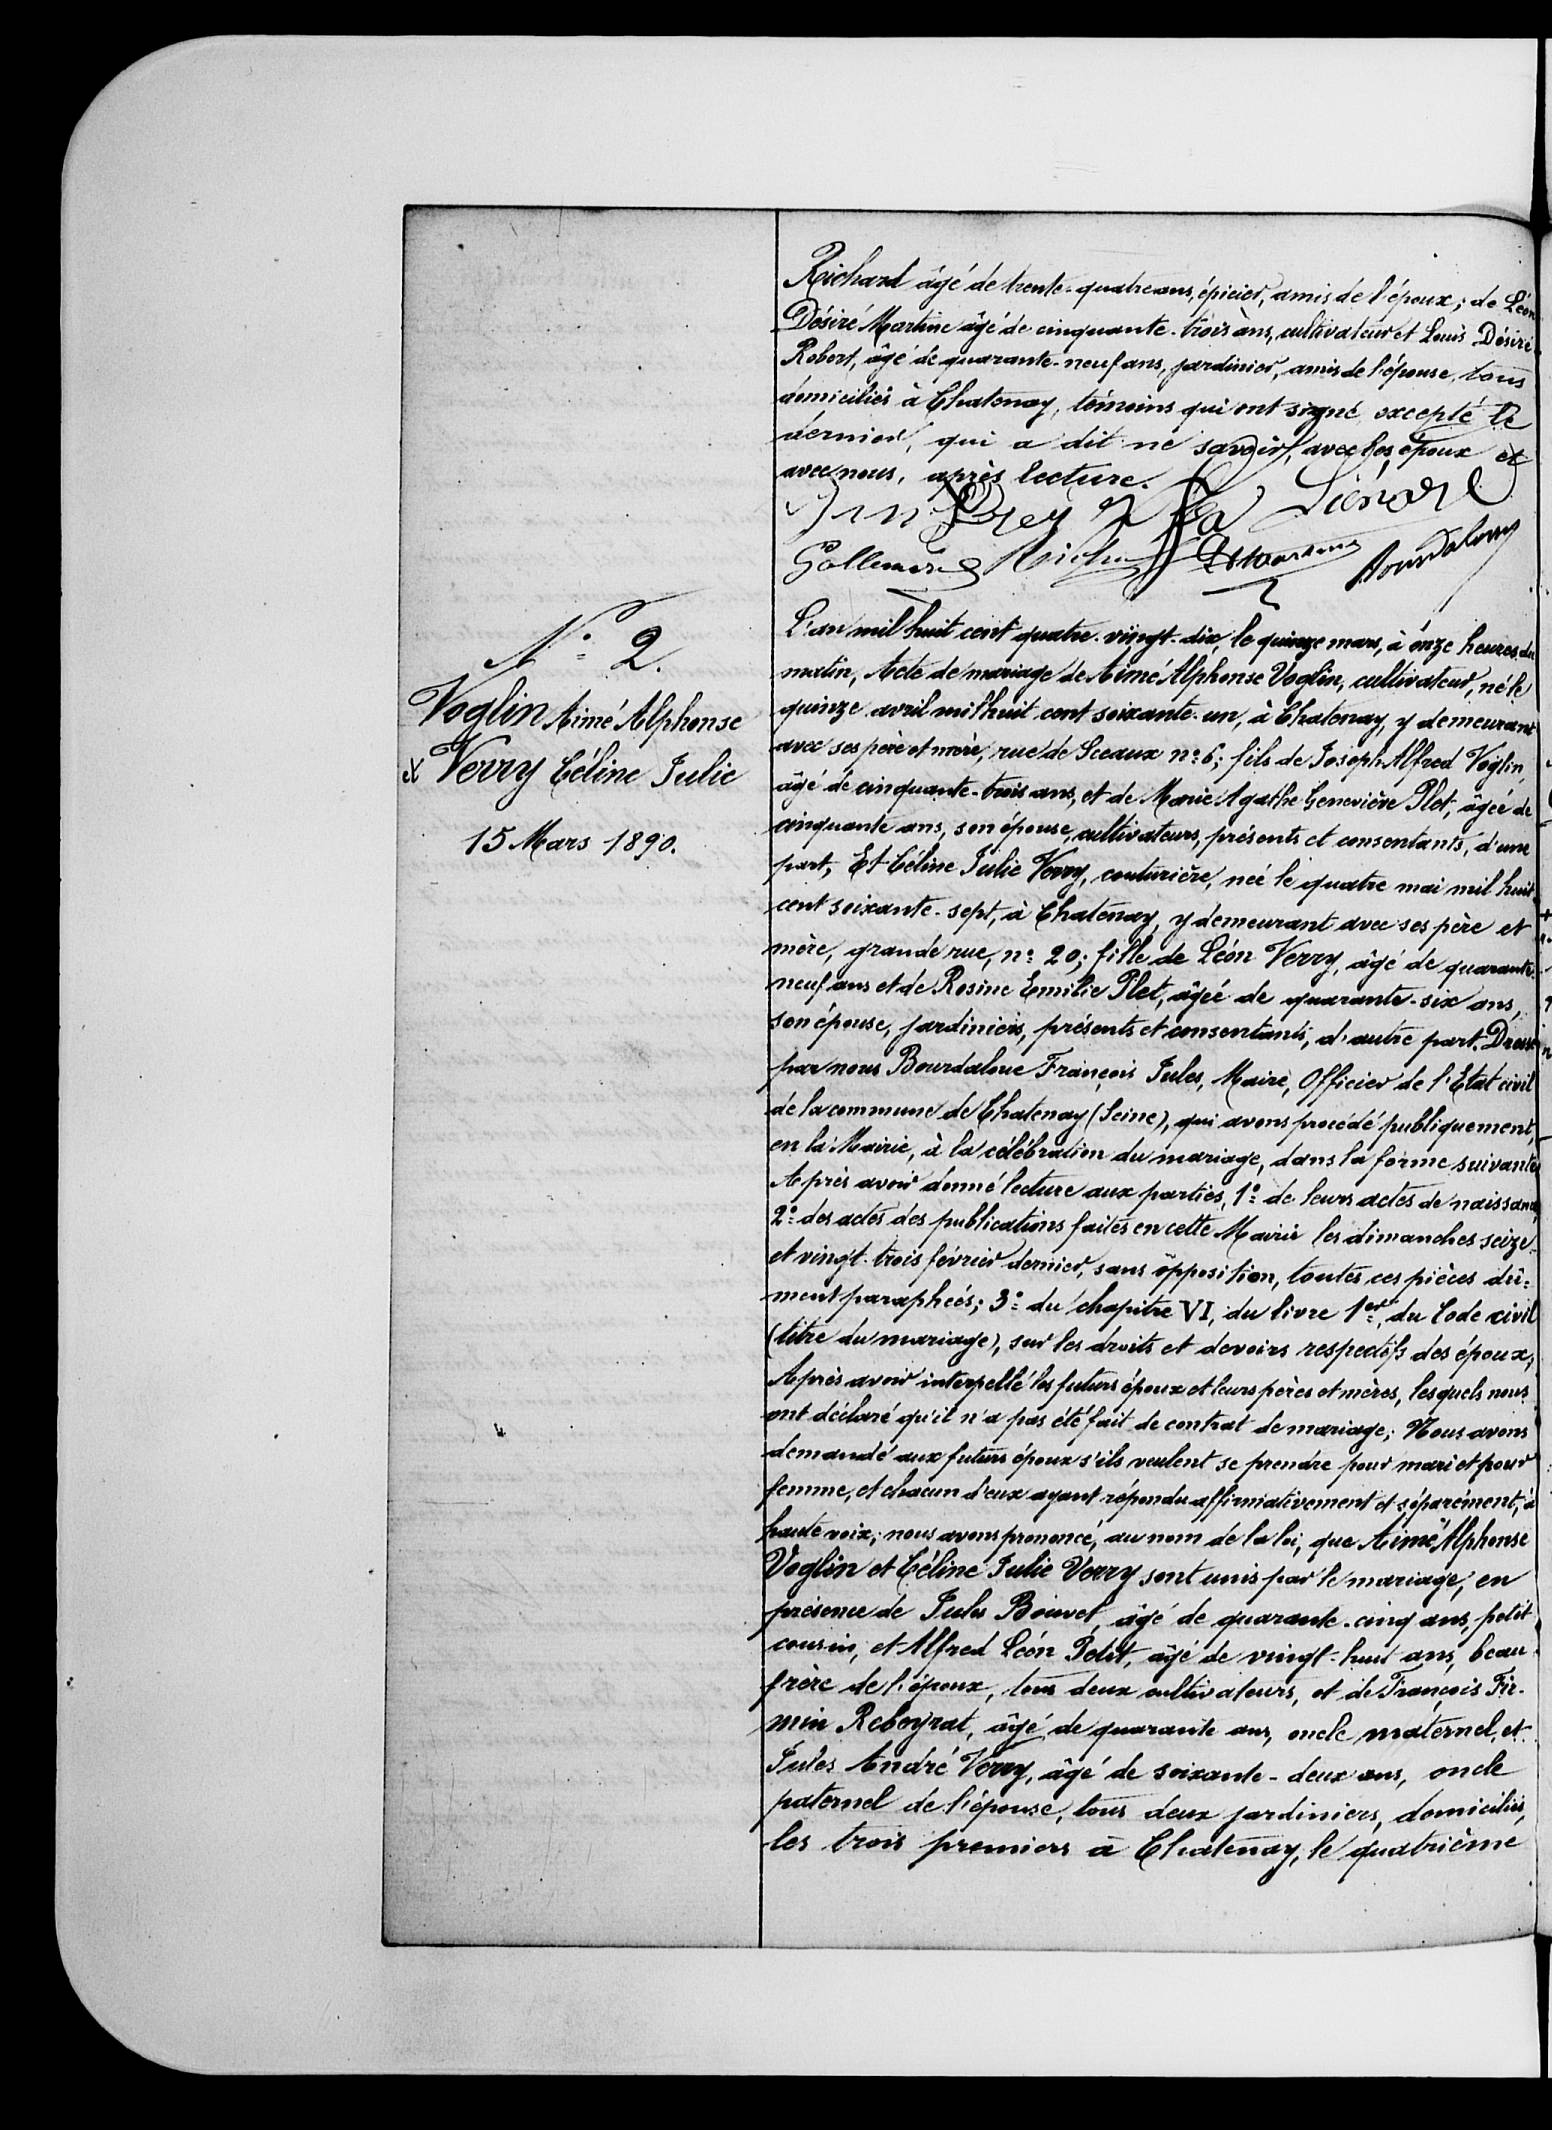Richard âgé de trentre-quatre ans, épicier, amis de l'époux; de Léon
Désiré Martine âgé de cinquante trois ans, cultivateur et Louis Désiré
Robert, âgé de quarante-neuf ans, jardinier, amis de l'épouse, tous
domiciliés à Chatonay, témoins qui ont signé accepté le
dernier, qui a dit ne savoir avec les époux et
avec nous, après lecture
N°2
Voglin Aimé Alphonse
et Verry Céline Julie
15 Mars 1890
L'an mil huit cent quatre vingt dix le quinze mars, à onze heures du
matin, Acte de mariage de Aimé Alphonse Voglin, cultivateur, né le
quinze avril mil huit cent soixante un, à Chatonay, y demeurant
avec ses père et mère, rue de Sceaux n°6; fils de Joseph Alfred Voglin,
âgé de cinquante-trois ans, et de Marie Agathe Geneviève Plot, âgée de
cinquante ans, son épouse, cultivateurs, présents et consentants, d'une
part, Et Céline Julie Verry, couturière, née le quatre mai mil huit
cent soixante-sept, à Chatenay, y demeurant avec ses père et
mère, grande rue, n°20; fille de Léon Verry, âgé de quarante
neuf ans et de Rosine Emilie Plet, âgée de quarante-six ans,
son épouse, jardinière, présents et consentants, d'autre part. Dressé
par nous Bourdaloue François Jules, Maire, Officier de l'Etat civil
de la commune de Chatenay (Seine), qui avons procédé publiquement
en la Mairie, à la célébration du mariage, dans la forme suivante
Après avoir donné lecture aux parties, 1:° de leurs actes de naissance
2:° des actes des publications faites en cette Mairie les dimanches seize
et vingt-trois février dernier, sans opposition, toutes ces pièces dû-
ment paraphées; 3:° du chapitre VI, du livre 1er dy Code civil
(titre du mariage) sur les droit et devoirs respectifs des époux
Après avoir interpellé les futurs époux et leurs pères et mères, lesquels nous
ont déclaré qu'il n'a pas été fait de contrat de mariage; Nous avons
demandé aux futurs époux s'ils veulent se prendre pour mari et pour
femme, et chacun d'eux ayant répondu affirmativement et séparément, à
haute voix; nous avons prononcé, au nom de a loi, quue Aimé Alphonse
Voglin et Céline Julie Verry sont unis par le mariage, en
présence de Jules Bouvel, âgé de quarante-cinq ans, petit
cousin, et Alfred Léon Petit, âgé de vingt-huit ans, beau
frère de l'époux, tous deux cultivateurs, et de François Fir-
min Rechoyrat, âgé de quarante ans, oncle maternel et
Jules André Verry, âgé de soixante-deux ans, oncle
paternel de l'épouse, tous deux jardinier, domiciliés,
les trois premiers à Chatenay, le quatrième
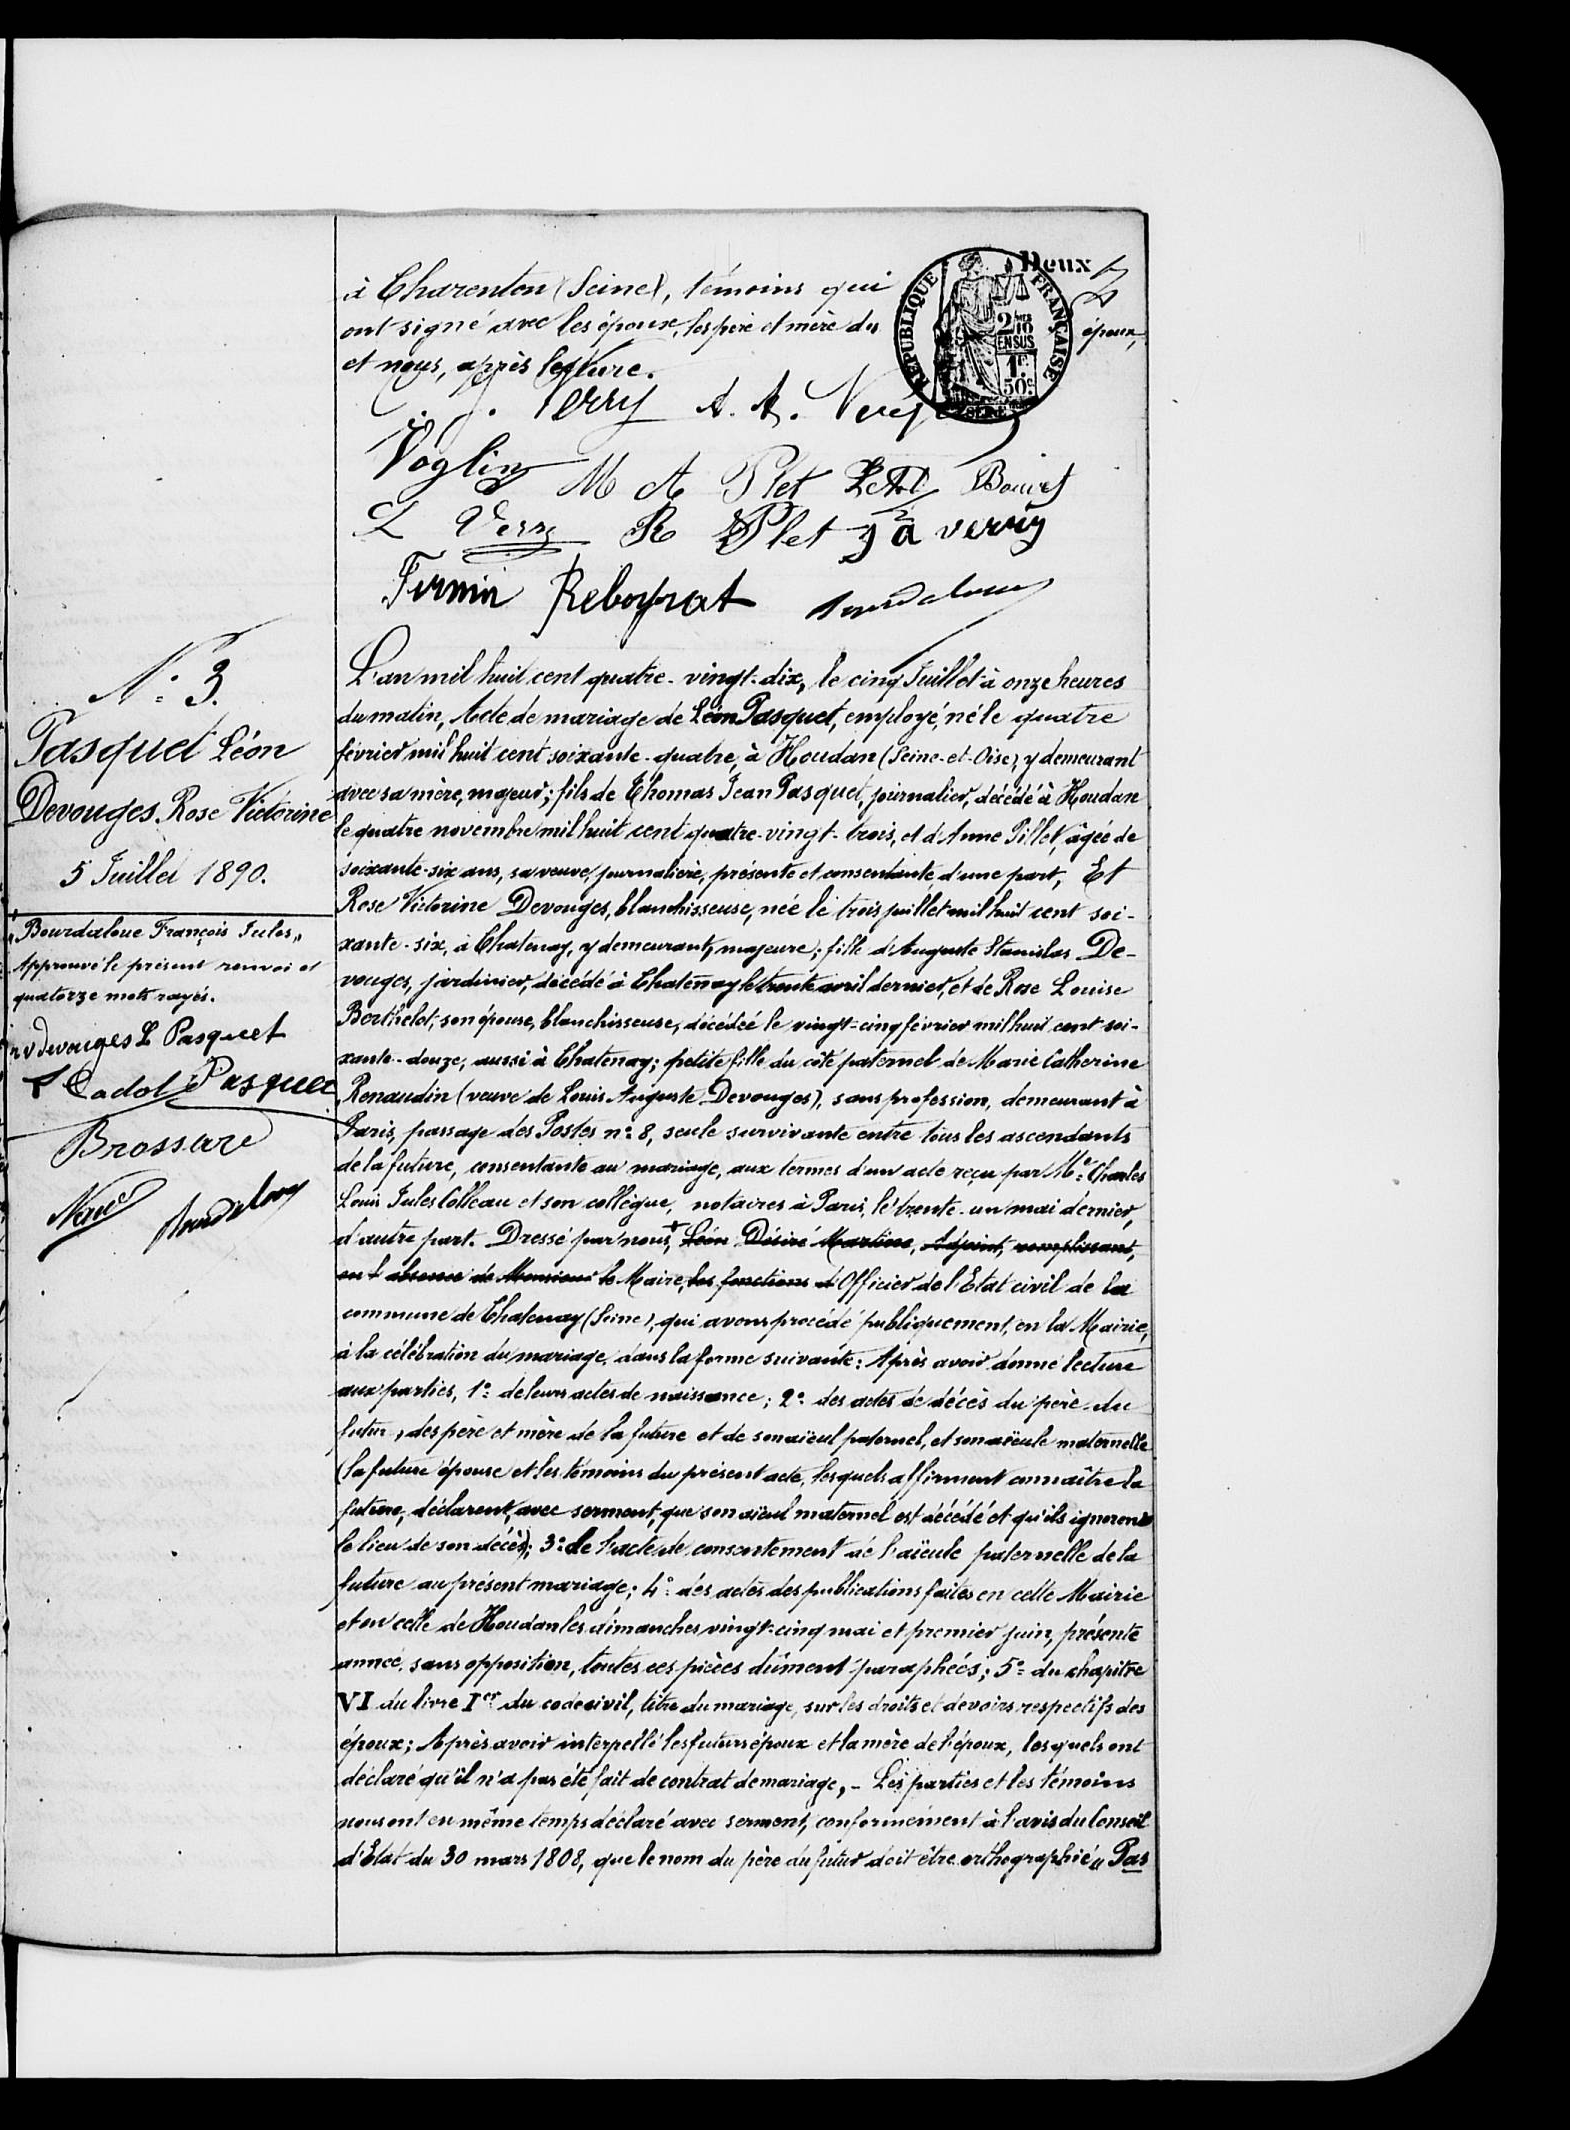à Charenton (Seine), témoins qui
ont signé avec les époux, les père et mère des époux,
et nous, après lecture.
N°3
Pasquet Léon
Devouges Rose Victorine
5 Juillet 1890
L'an mil huit cent quatre-vingt-dix, le cinq Juillet à onze heures
du matin, Acte de mariage de Léon Pasquet, employé, né le quatre
février mil huit cent soixante-quatre, à Houdan (Seine-et-Oise), y demeurant
avec sa mère, majeur; fils de Thomas Jean Pasquet, journalier, décédé à Houdan
le quatre novembre mil huit cent quatre-vingt-trois, et d'Anne Pillot, âgée de
soixante-six ans, serveuse, journalière, présente et consentante d'une part, Et
Rose Victorine Devouges, blanchisseuse, née le trois juillet mil huit cent soi-
xante-six, à Chatenay, y demeurant, majeure; fille d'Auguste Stanislas De-
vouges, jardinier, décédé à Chatenay le trente avril dernier, et de Rose Louise
Berthelot, son épouse, blanchisseuse, décédée le vingt-cinq février mil huit cent soi-
xante-douze; aussi à Chatenay; petite fille du côté paternel de Marie Catherine
Renaudin (veuve de Louis Auguste Devouges), sans profession, demeurant à
Paris, passage des Postes n° 8, seule survivante entre tous les ascendants
de la future, consentante au mariage, aux termes d'un acte reçu par Me Charles
Louis Jules Colleau et son collègue, notaires à Paris, le trente-un mai dernier,
d'autre part. Dressé pour nous, Léon Désiré Martin, Adjoint, remplissant
en l'absence de Monsieur le Maire, les fonctions d'Officier de l'Etat civil de la
commune de Chatenay (Seine), qui avons procédé publiquement, en la Mairie,
à la célébration du mariage dans la forme suivante: Après avoir donné lecture
aux parties, 1°: de leurs actes de naissance; 2°: des actes de décès du père du
futur, des père et mère de la future et de son aïeul paternel, et son aïeule maternelle
la future épouse et les témoins du présent acte, lesquels affirment connaître la
future, déclarent avec serment que son aïeul maternel est décédé et qu'ils ignorent
le lieu de son décès); 3°: de l'acte de consentement de l'aïeule paternelle de la
future au présent mariage; 4° des actes des publications faites en cette Mairie
et en celle de Houdan les dimanches vingt-cinq mai et premier juin, présente
année, sans opposition, toutes ces pièces dûment paraphées; 5° du chapitre
VI du livre Ier du code civil, titre du mariage, sur les droits et devoirs respectifs des
époux; Après avoir interpellé les futurs époux et la mère de l'époux, lesquels ont
déclaré qu'il n'a pas été fait de contrat de mariage,-Les parties et les témoins
nous ont en même temps déclaré avec serment, conformément à l'avis du Conseil
d'Etat du 30 mars 1808, que le nom du père du futur doit être orthographié "Pas